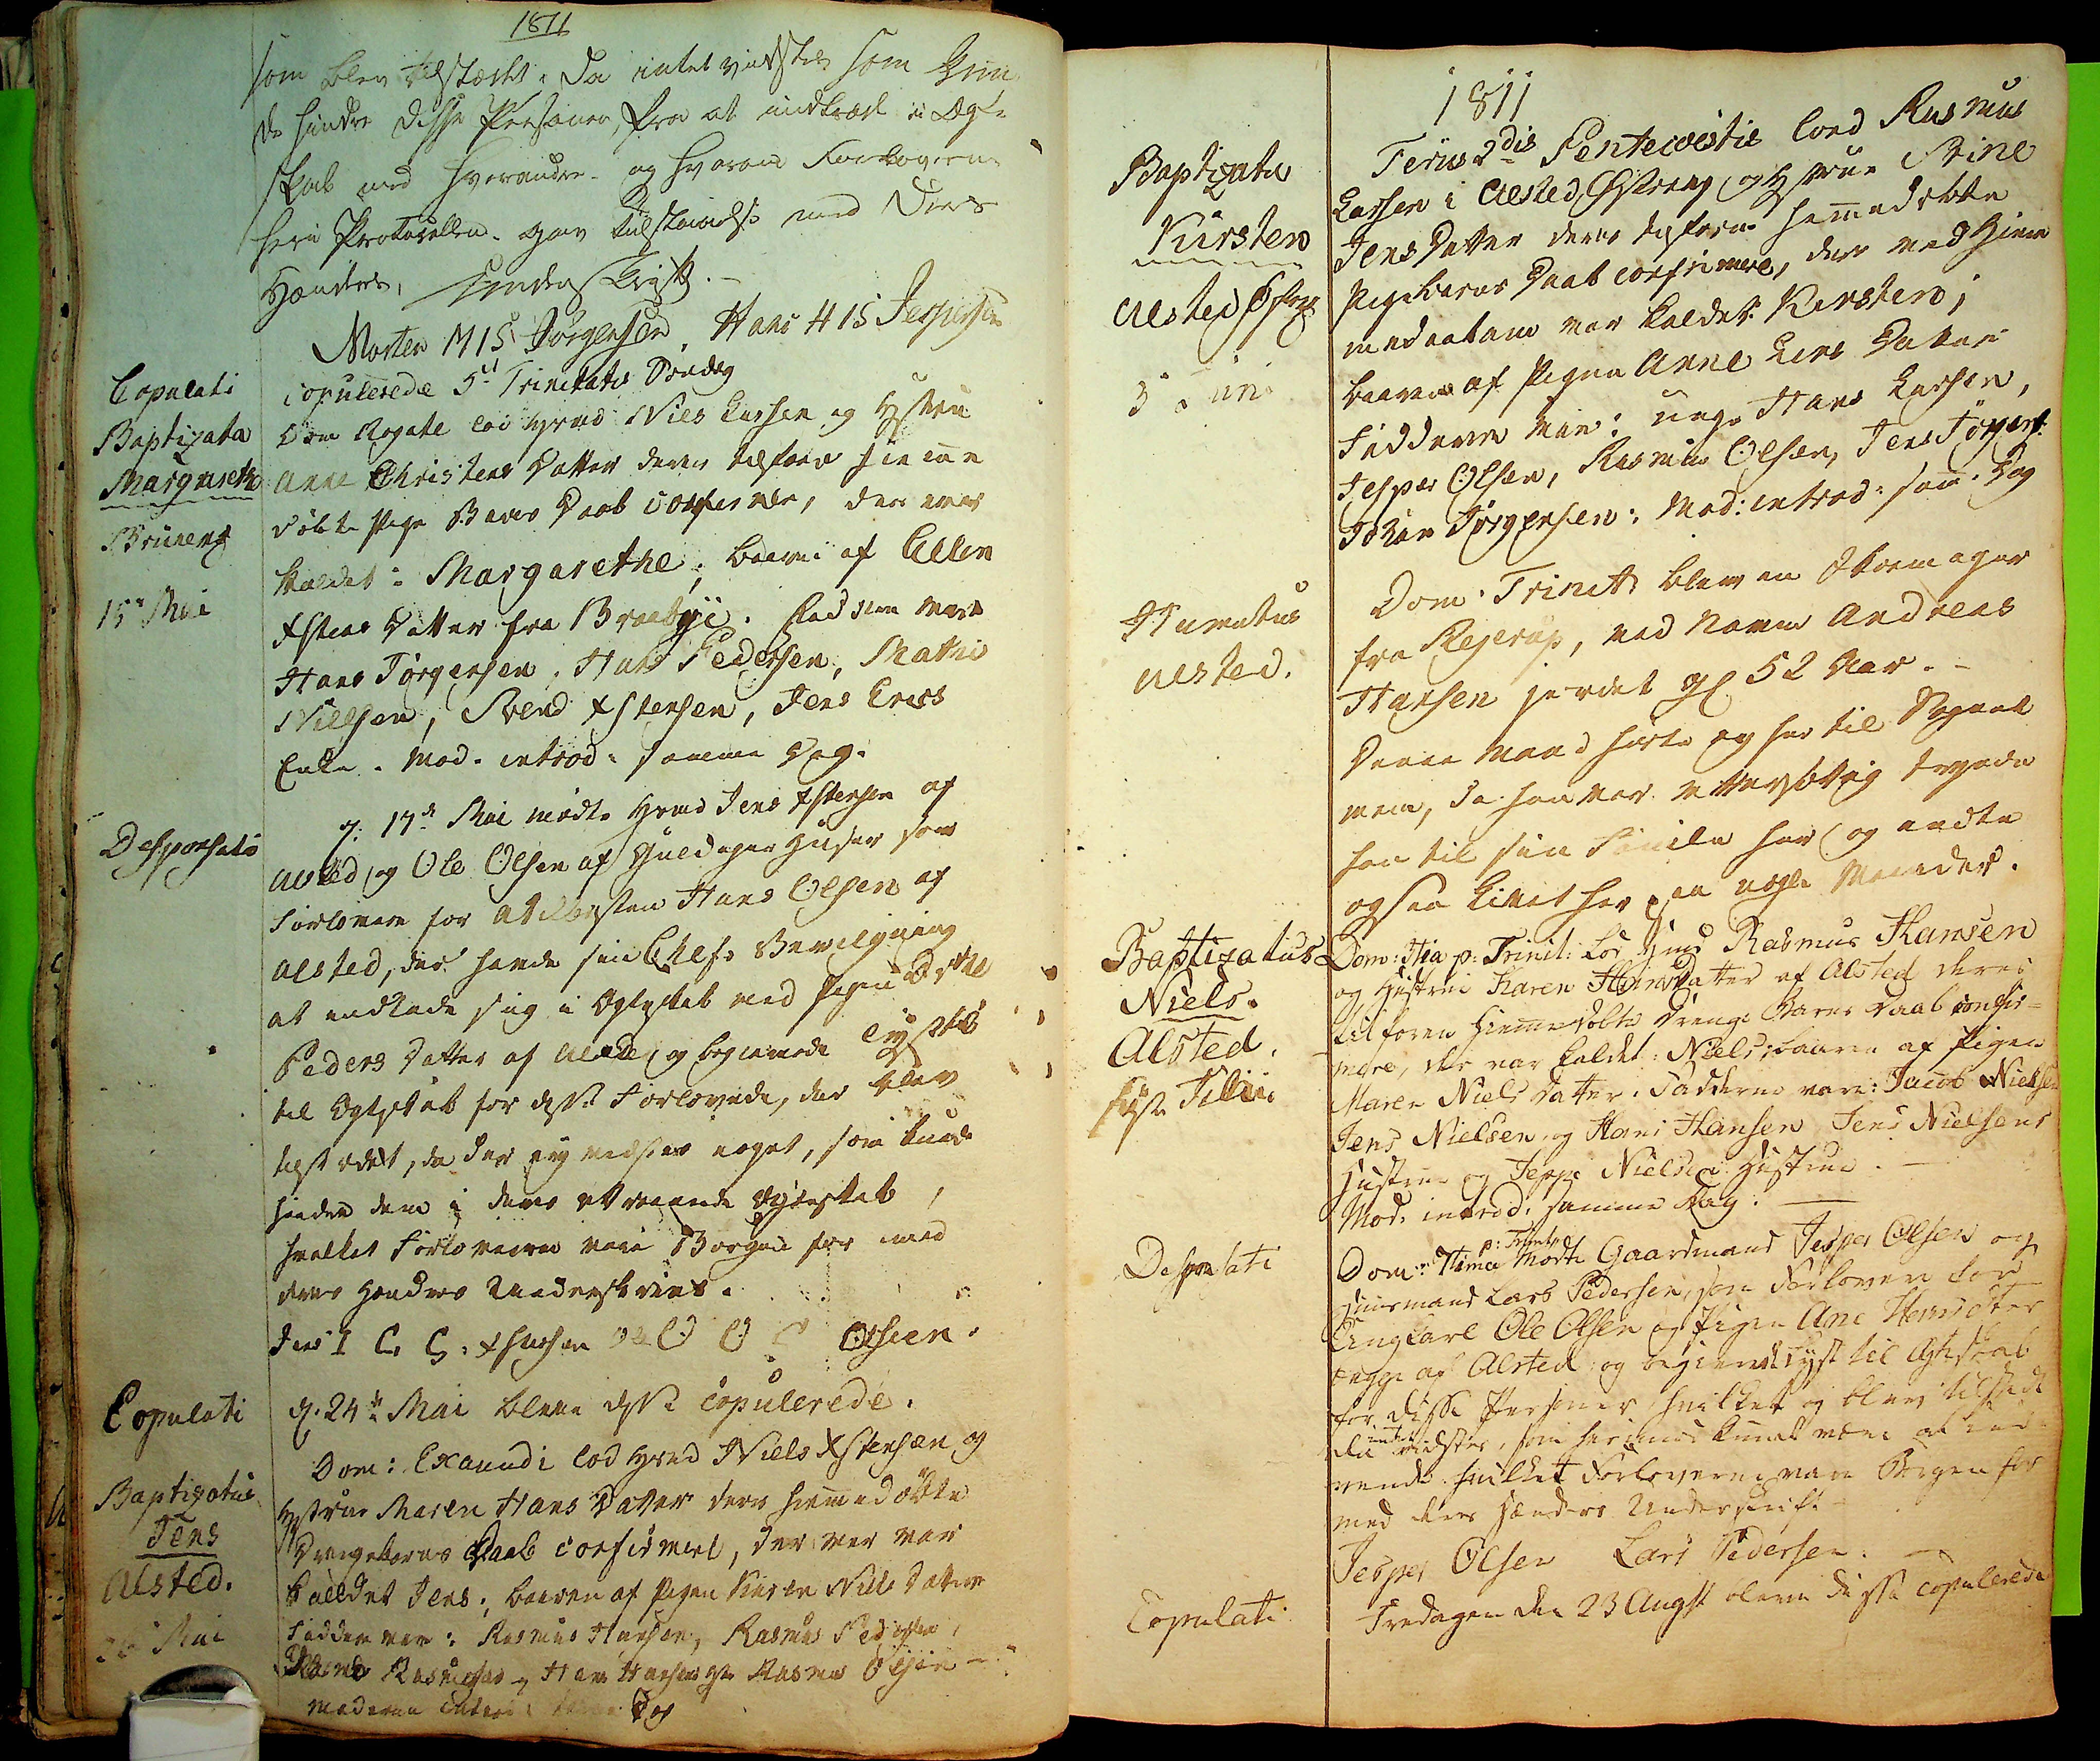Copulati
Baptizata
Margrethe
Brunem##
15## Mai
Desponsati
Copulati
Baptizatus
Jens
Alsted.
26 Mai
1811
som blev tilstædet da intet vidste som kun
de hindre disse Personer, fra at indræde i Ægte¬
skab med hverande og hvorved Forloverne
heri Protovellen gav tilsta##lse med deres
Hænders Underskrift.
Morten M I S Jørgensøn Hans H I S Jespersen
copulerede 5## Trinitatis Søndag
Dom Rogate lod Grmd Niels Lassen og Hstru
Anne Christens Datter deres tilforn hiemme
døbt Pige Barns Daab confirmere, der var
kaldet: Margarethe. baaren af Ellen
Xstens Datter fra Braabye. Faddere vare
Hans Jørgensen, Hans Pedersen, Mathis
Nielsen, Svend Xstensen, Jens Erics
Enke. Mod. introd: samme Dag.
d: 17de Mai mødte Hsmd Jens Xstensen af
Alsted og Ole Olsen af Guldager Huset som
Forlovere for atilleristen Hans Olsen af
Alsted, der havde sin Chefs Bevilgning
at indlade sig i Ægteskab med Pigen Dorthe
Peders Datter af Alsted og begierede lyst
til Ægteskab for disse Forlovede, der blev
tilstædet, da der ey vidstes noget, som kunde
hindre dem i deres attraaede Ægteskab
hvilket Forloverne vare Borgen for med
deres Hænders Underskrivt.
Jens I C S Xstensen Ole O O S Olsen.
d. 24de Mai bleve disse copulerede.
Dom: Exaudi lod Hsmd Niels Xstensen og
Hstrue Maren Hans Datter deres hiemmedøbte
Drengebarns Daab confirmere, der var var
kaldet Jens. baaren af Pigen Kirsten Niels Datter
Faddere vare: Rasmus Hansen, Rasmus Pedersen
Rasmus Rasmusens og Hans Hansens Hstr Rasmus Olsen
Moderen introd: samme Dag
Bapizata
Kirsten
Alsled Østrem##
3## Jun
Humatus
Alsted
Baptizatus
Niels
Alsted
1ste Julii
Desponsati
Copulati.
1811
Feriis 2dis Pentecostis loed Rasmus
Larsen i Alsted Østrem## og Hstrue Stine
Jens Datter deres tilforn hiemmedøbte
Pigebarns Daab Confirmere, der ved Hiem
medaaben var kaldet Kirsten;
baaren af Pigen Anne Lars Datter
Fadderne var: ung Hans Larsen,
Jesper Olsen, Rasmus Olsen, Jens Jørgens:
Johan Jørgensen: Mod introd: samme Dag
Dom. Trinit blev en Skoemager
fra Rejerup, ved Navn Andreas
Hansen jordet gl 52 Aar.
Denne Mand horte ey her til Sognet
men, da han var ## ##
han til sin F##le har og aadte
ogsaa Livet her paa nogle M##
Dom: 3tia p: Trinit: Lod Hmd Rasmus Hansen
og Hustrue Karen Hansdatter af Alsted deres
tilforn hiemmedøbte Drenge Barns Daab confir¬
mere, der var kaldet: Niels, baaren af Pigen
Maren Niels Datter. Fadderne vare: Jacob Nielsen
Jens Nielsen og Hans Hansen, Jens Nielsens
Hustrue og Jeppe Nielsens Hustrue
Mod introd: samme Dag.
p: Trint
Dom: 7tima Mødte Gaardmand Jesper Olsens og
Huusmand Lars Pedersen, som Forlovere for
Ungkarl Ole Olsen og Pigen Ane Hansdatter
begge af Alsted og begierede lyst til Ægteskab
for disse Personer, hvilket og blev tilstædet
intet
da vidstes, som herimod kunde være at ind¬
vende hvilket Forloverne vare Borgen for
med deres Hænders Underskrift.
Jesper Olsen Lars Pedersen
Fredagen den 23 Augst bleve disse copulerede
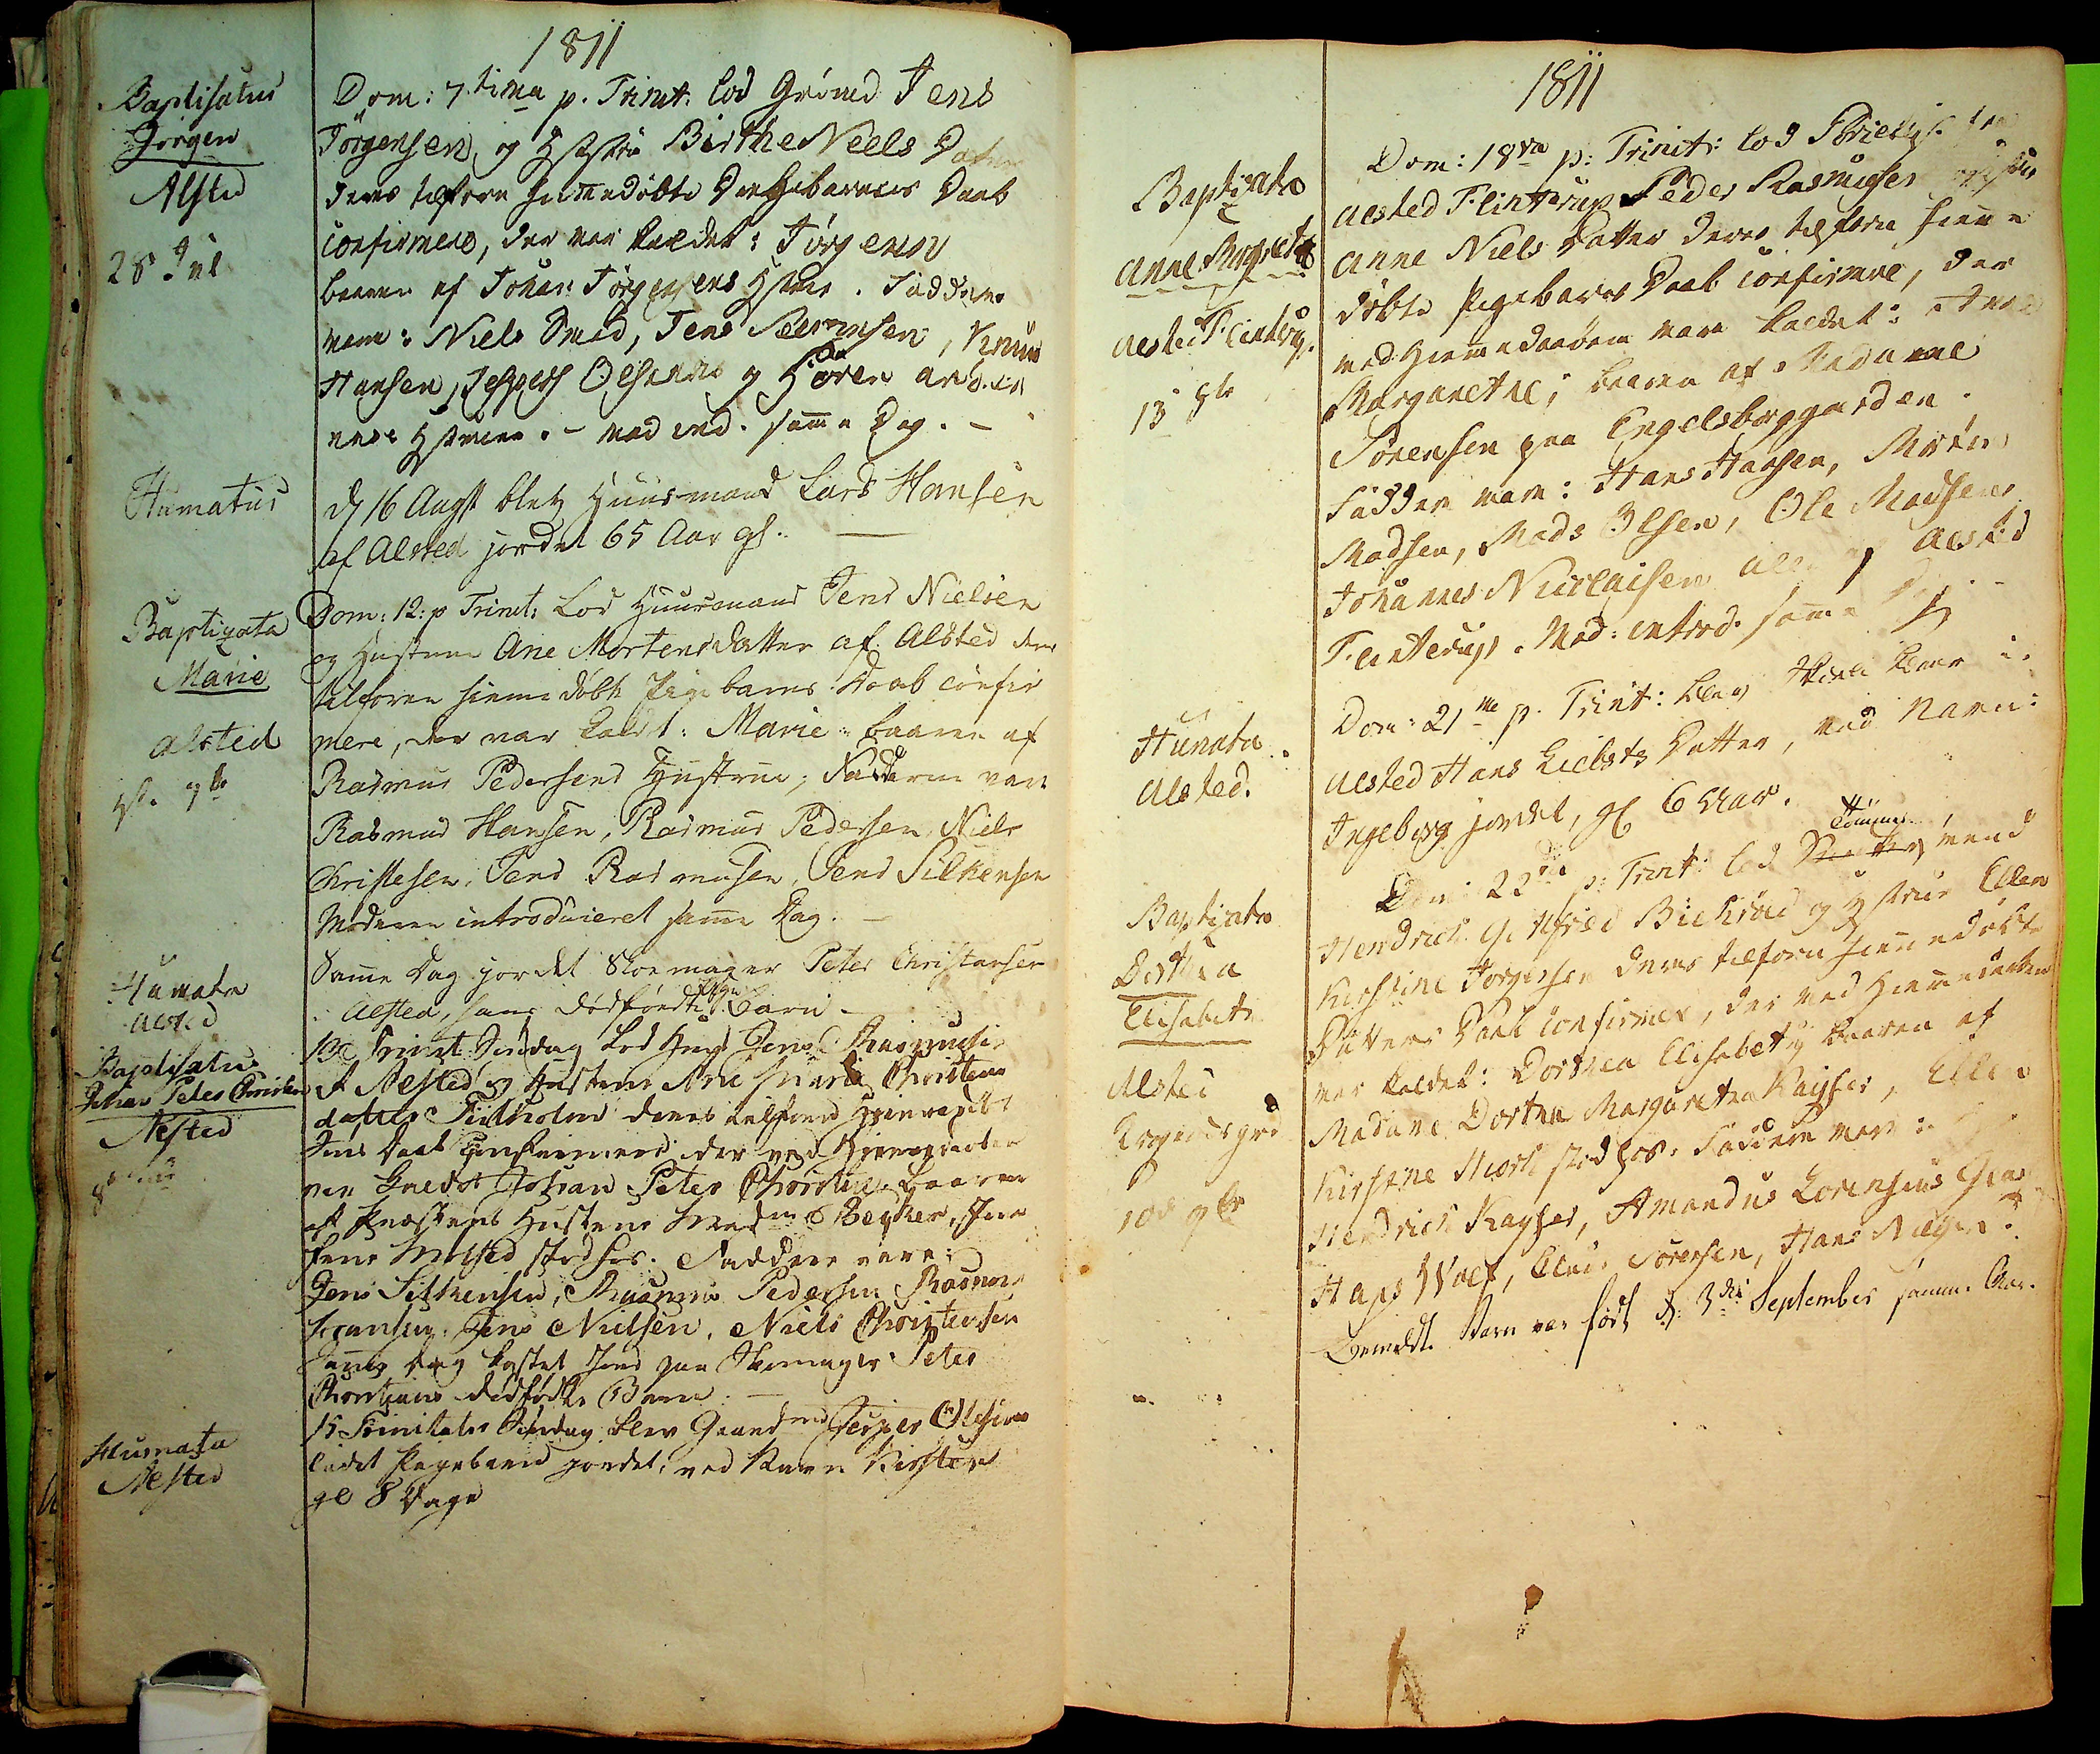Baptisatus
Jørgen
Olsted
28 Jul
Humatus
Baptizata
Marie
Alsted
1ste 7br
Humata
Alsted
BaptisatusRasliati
Johan Peder Christen
Alsted
8de Septbr
Humata
Alsted
1811
Dom: 7tima p. Trinit: lod Grdmd Jens
Jørgensen, og Hstru Birthe Neels Dater
deres tilforn hiemedøbte Drengebarns Daab
confirmere, der var kaldet: Jørgen
baaren af Johan Jørgensens Hstru. Faddere
vare Niels Smid, Jens Selkensen, Knud
Hansen Jeppe Olsens og Søren Ander
sens Hstruer. - Mod ind: samme Dag.
d 16 Augst blev Huusmand Lars Hansen
af Alsted jordet 65 Aar gl:
Dom: 12: p Trinit: Lod Huusmand Jens Nielsen
og Hustrue Anne Mortensdatter af Alsted den
tilforn hieme døbte Pigebarn. Daab confir
mere, der var kaldt: Marie, baaren af
Rasmus Pedersens Hustrue; Fadderne vare:
Rasmus Hansen, Rasmus Pedersen, Niels
Christensen, Jens Rasmusen, Jens Silkensen
Moderen introduceret samme Dag
Same Dag jordet Skoemager Peter Christensen
Pige
i Alster, hans dødføedte Barn
13 Trinit: Søndag Lod Jens Jens Rasmussen
af Alsted og Hustrue Anne Marie Christen
datter Fiitholm deres tilforn Hiemmedøbte
Søns Daab Conformere der ved Hiemedaaben
var kaldet Johan Peter Chistian baaren
af Præstens Hustrue Madm Becher, Jm
frue Mølsted stod hos. Faddere vare:
Jens Silkensen, Rasmus Pedersen Rasmus
Hansen, Jens Nielsen, Niels Christensen
Samme Dag kastet Jord paa Skomager Peter
Christensens dødfødte Barn
15 Trinitatis Søndag blev Gaardmd Jesper Olesens
lidet Pigebard jordet ved Navn Kirsten
d 8 Dage.
Baptizata
Anne Margrethe
Alsted Flinterup
13## 8br
Humata
Alsted.
Batizata
Dorthea
Elisabeth
Alsted
Kroge##grd
10de 9br
1811
Dom: 18va p: Trinit: lod F## fra
Alsted Flinterup Peder Rasmussen og Hstr
Anne Niels Datter deres tilforn hiemme
døbte Pigebarns Daab confirme, der
ved Hiemmedaaben var kaldet: Anne
Margarethe, baaren af Madame
Sørensen paa Engelsborggaarden.
Fadder vara: Hans Hansen, M##
Madsen, Mads Olsen, Ole Madsen
Johannes Niculaisen alle af Alsted
Flinterup. Mod: introd: samme Dag
Dom: 21## p: Trinit: blev Skole Lærer i
Alsted Hans Liebsts Datter, ved Navn:
Ingeborg jordet, gl: 6 Aar.
Tømmer
Den 22## p: Trint: lod Sneker mand
Hendrich Gottfried Biehrøed og Hstrue Ellen
Kirstine Jørgensen, deres tilforn hiemmedøbte
Datters Daab confirmere, der ved Hiemmedaaben
var kaldet: Dorthea Elisabet, baaren af
Madame Dorthea Margaretha Kayser, Ellen
Kirstine Hiort stod hos. Fadderne vare: Hr
Hendrich Kayser, Amandus Lorensens G##
Hans Wolf, Claus Sørensen, Hans Nilsen.
Bemeldte Barn var født d: 3die September samme Aar.